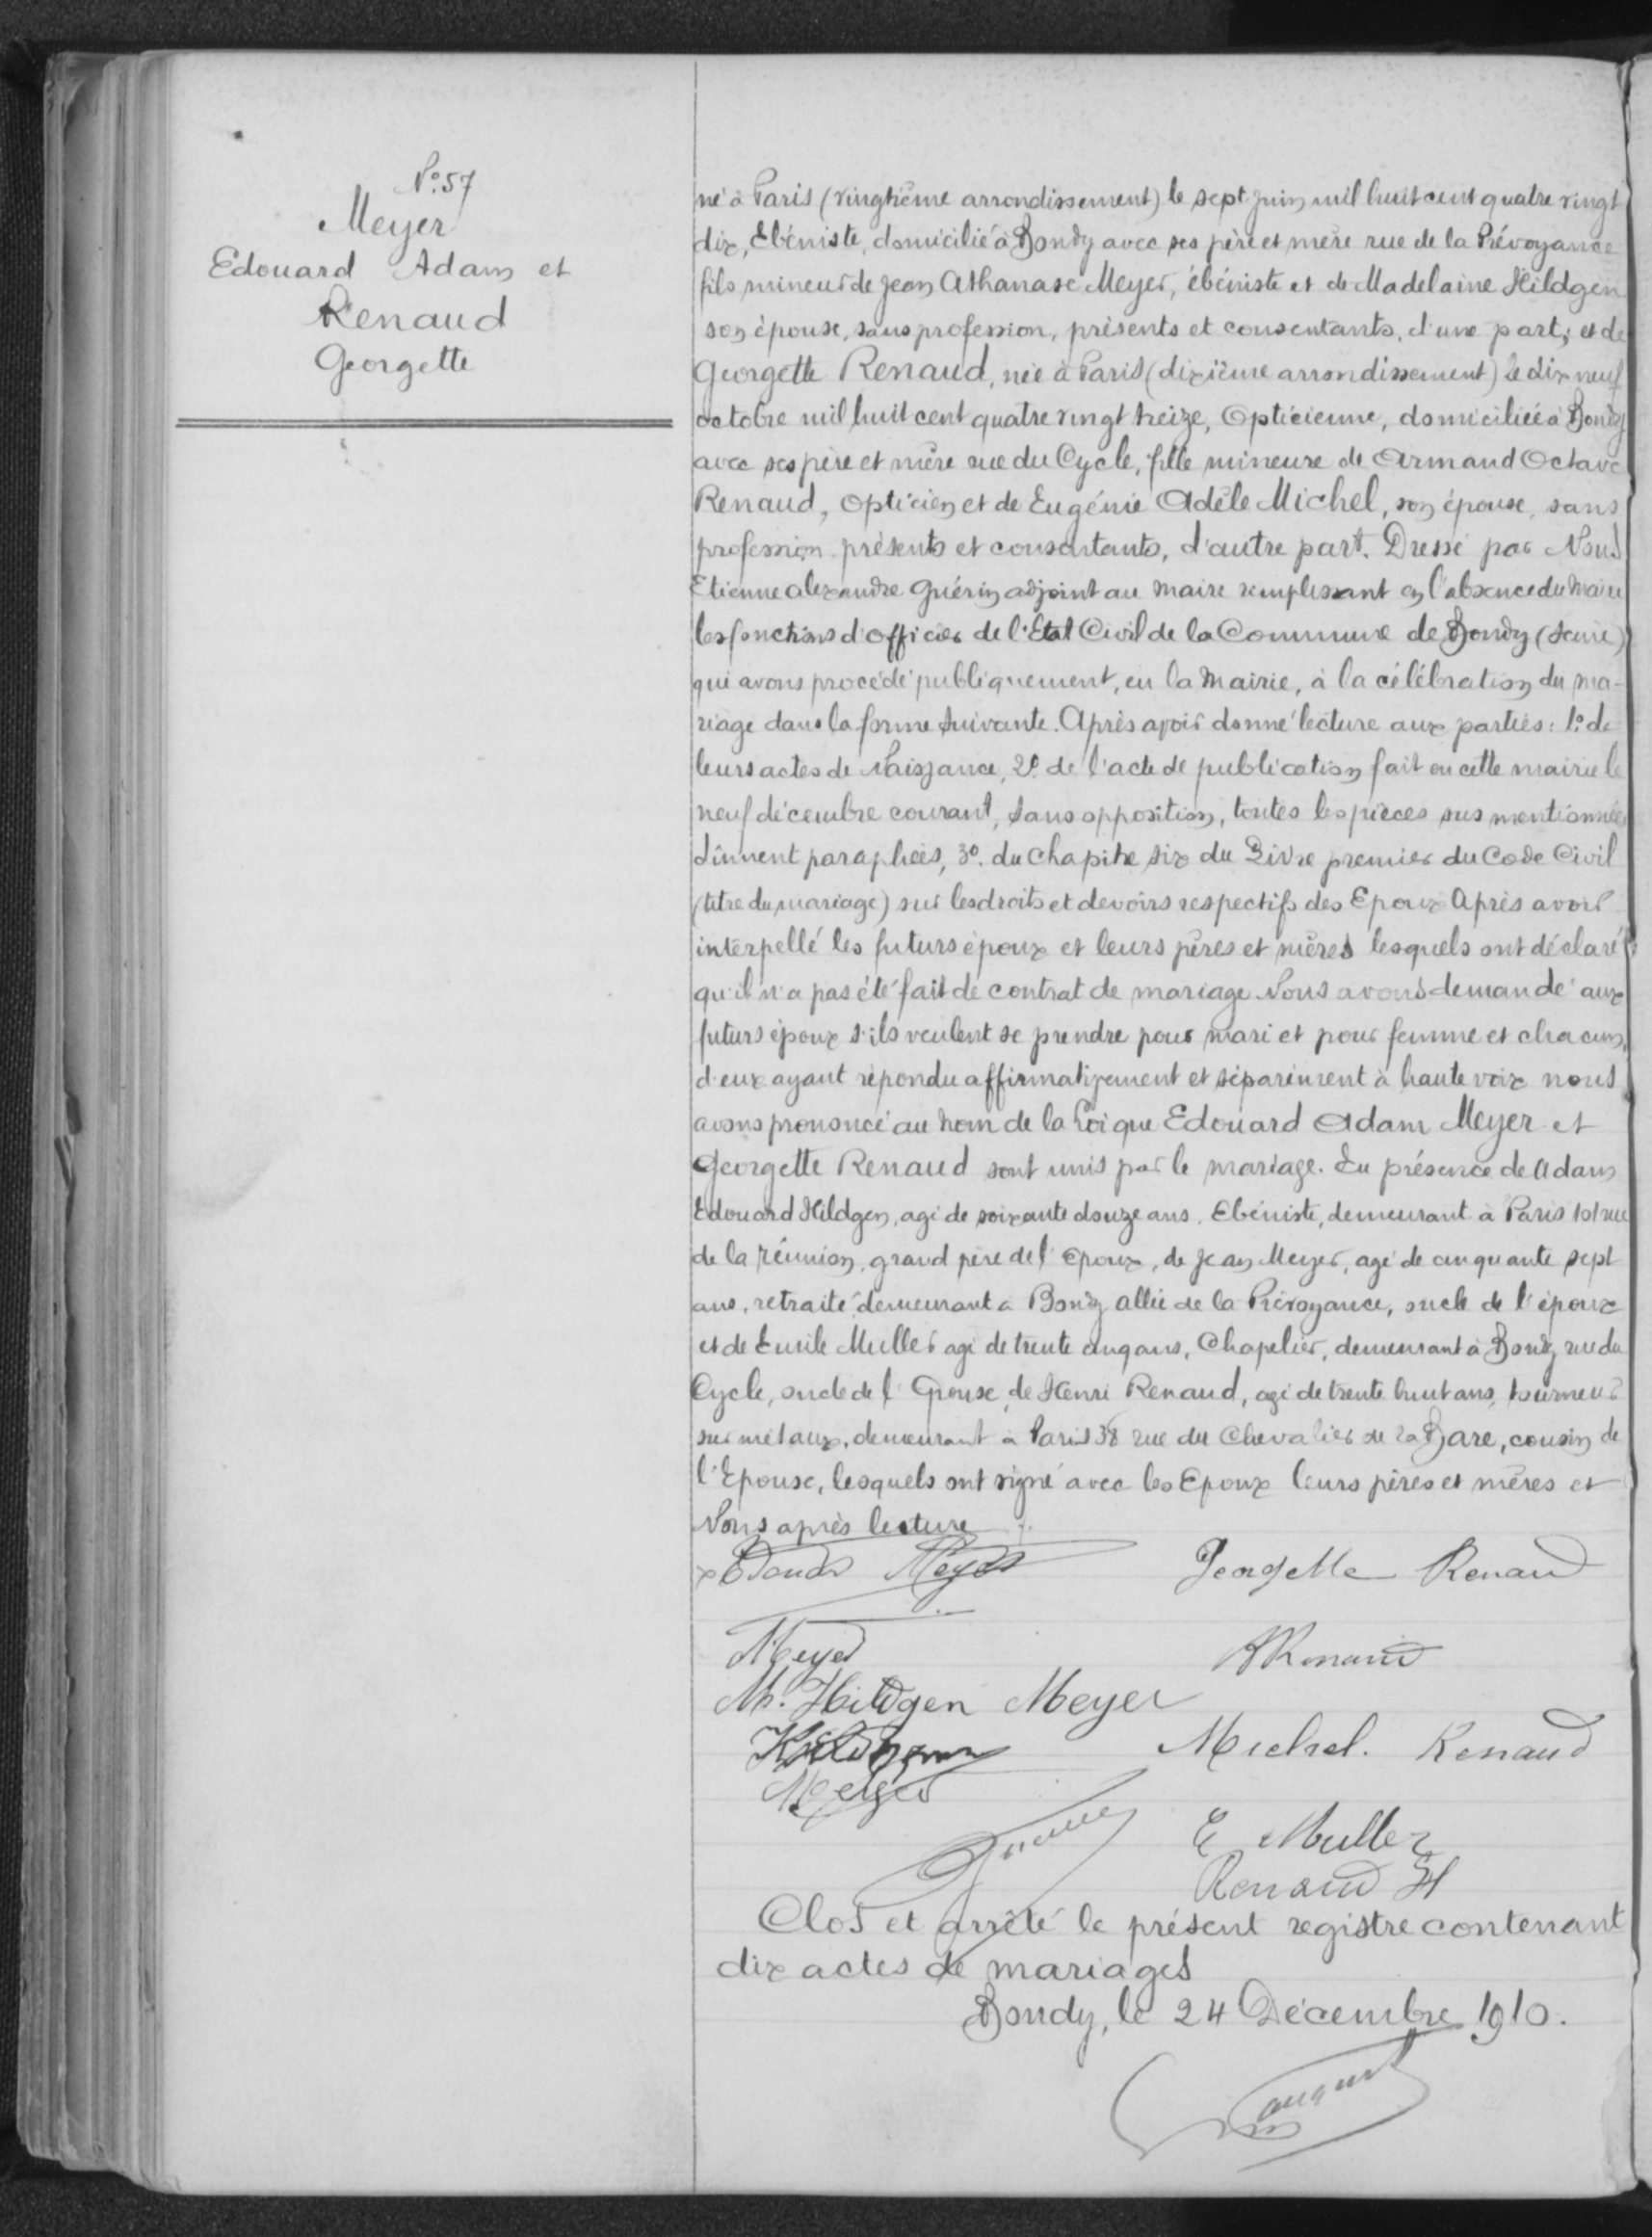N°57
Meyer
Edouard Adam et
Renaud
Georgette
né à Paris (vingtième arrondissement) le sept juin mil huit cent quatre vingt
dix Ebéniste, domicilié à Bondy avec ses père et mère rue de la Prévoyance
fils mineur de Jean Athanase Meyer, ébéniste et de Madelaine Hildgen
son épouse, sans profession, présents et consentants, d'une part; et de
Georgette Renaud, née à Paris (dixième arrondissement) le dix neuf
octobre mil huit cent quatre vingt treize, opticienne, domiciliée à Bondy
avec ses père et mère rue du Cycle, fille mineure de Armand Octave
Renaud, opticien et de Eugénie Adèle Michel, son épouse, sans
profession, présents et consentants, d'autre part. Dressé par Nous
Etienne Alexandre Guérin adjoint au maire remplissant en l'absence du Maire
les fonctons d'Officier de l'Etat Civil de la commune de Bondy (Seine)
qui avons procédé publiquement en la Mairie, à la célébration du ma-
riage dans la forme suivante. Après avoir donné lecture aux parties 1°: de
leurs actes de naissance, 2°: de l'acte de publications fait en cette mairie le
neuf décembre courant sans opposition, toutes les pièces sus mentionnées
dûment paraphées, 3° du Chapitre six du Livre premier du Code Civil
(titre du mariage) sur les droits et devoirs respectifs des Epoux après avoir
interpellé les futurs époux et leurs pères et mrèes lesquels ont déclaré
qu'il n'a pas été fait de contrat de mariage Nous avons demandé aux
futurs époux s'ils veulent se prendre pour mai et pour femme et chacun
d'eux ayant répondu affirmativement et séparément à haute voix nous
avons prononcé au nom de la Loi que Edouard Adam Meyer et
Georgette Renaud sont unis par le mariage. En présence de Adam
Edouard Hildgen, agé de soixante douze ans, ebéniste, demeurant à Paris 101 rue
de la réunion, grand père de l'Epoux, de Jean Meye, agé de cinquante sept
ans, retraité demeurant à Bondy allée de la Prévoyance, oncle de l'époux
et de Emile Mull agé de trente cinq ans, Chapelier, demeurant à Bondy, rue du
Cycle, oncle de l'Epouse, de Henri Renaud, agé de trente huit ans, tourneur
sur métaux, demeurant à Paris 38 rue du Chevalier de la Gare, cousin de
l'Epouse, lesquels ont signé avec les Epoux leurs pères et mères et
Nous après lecture ./.